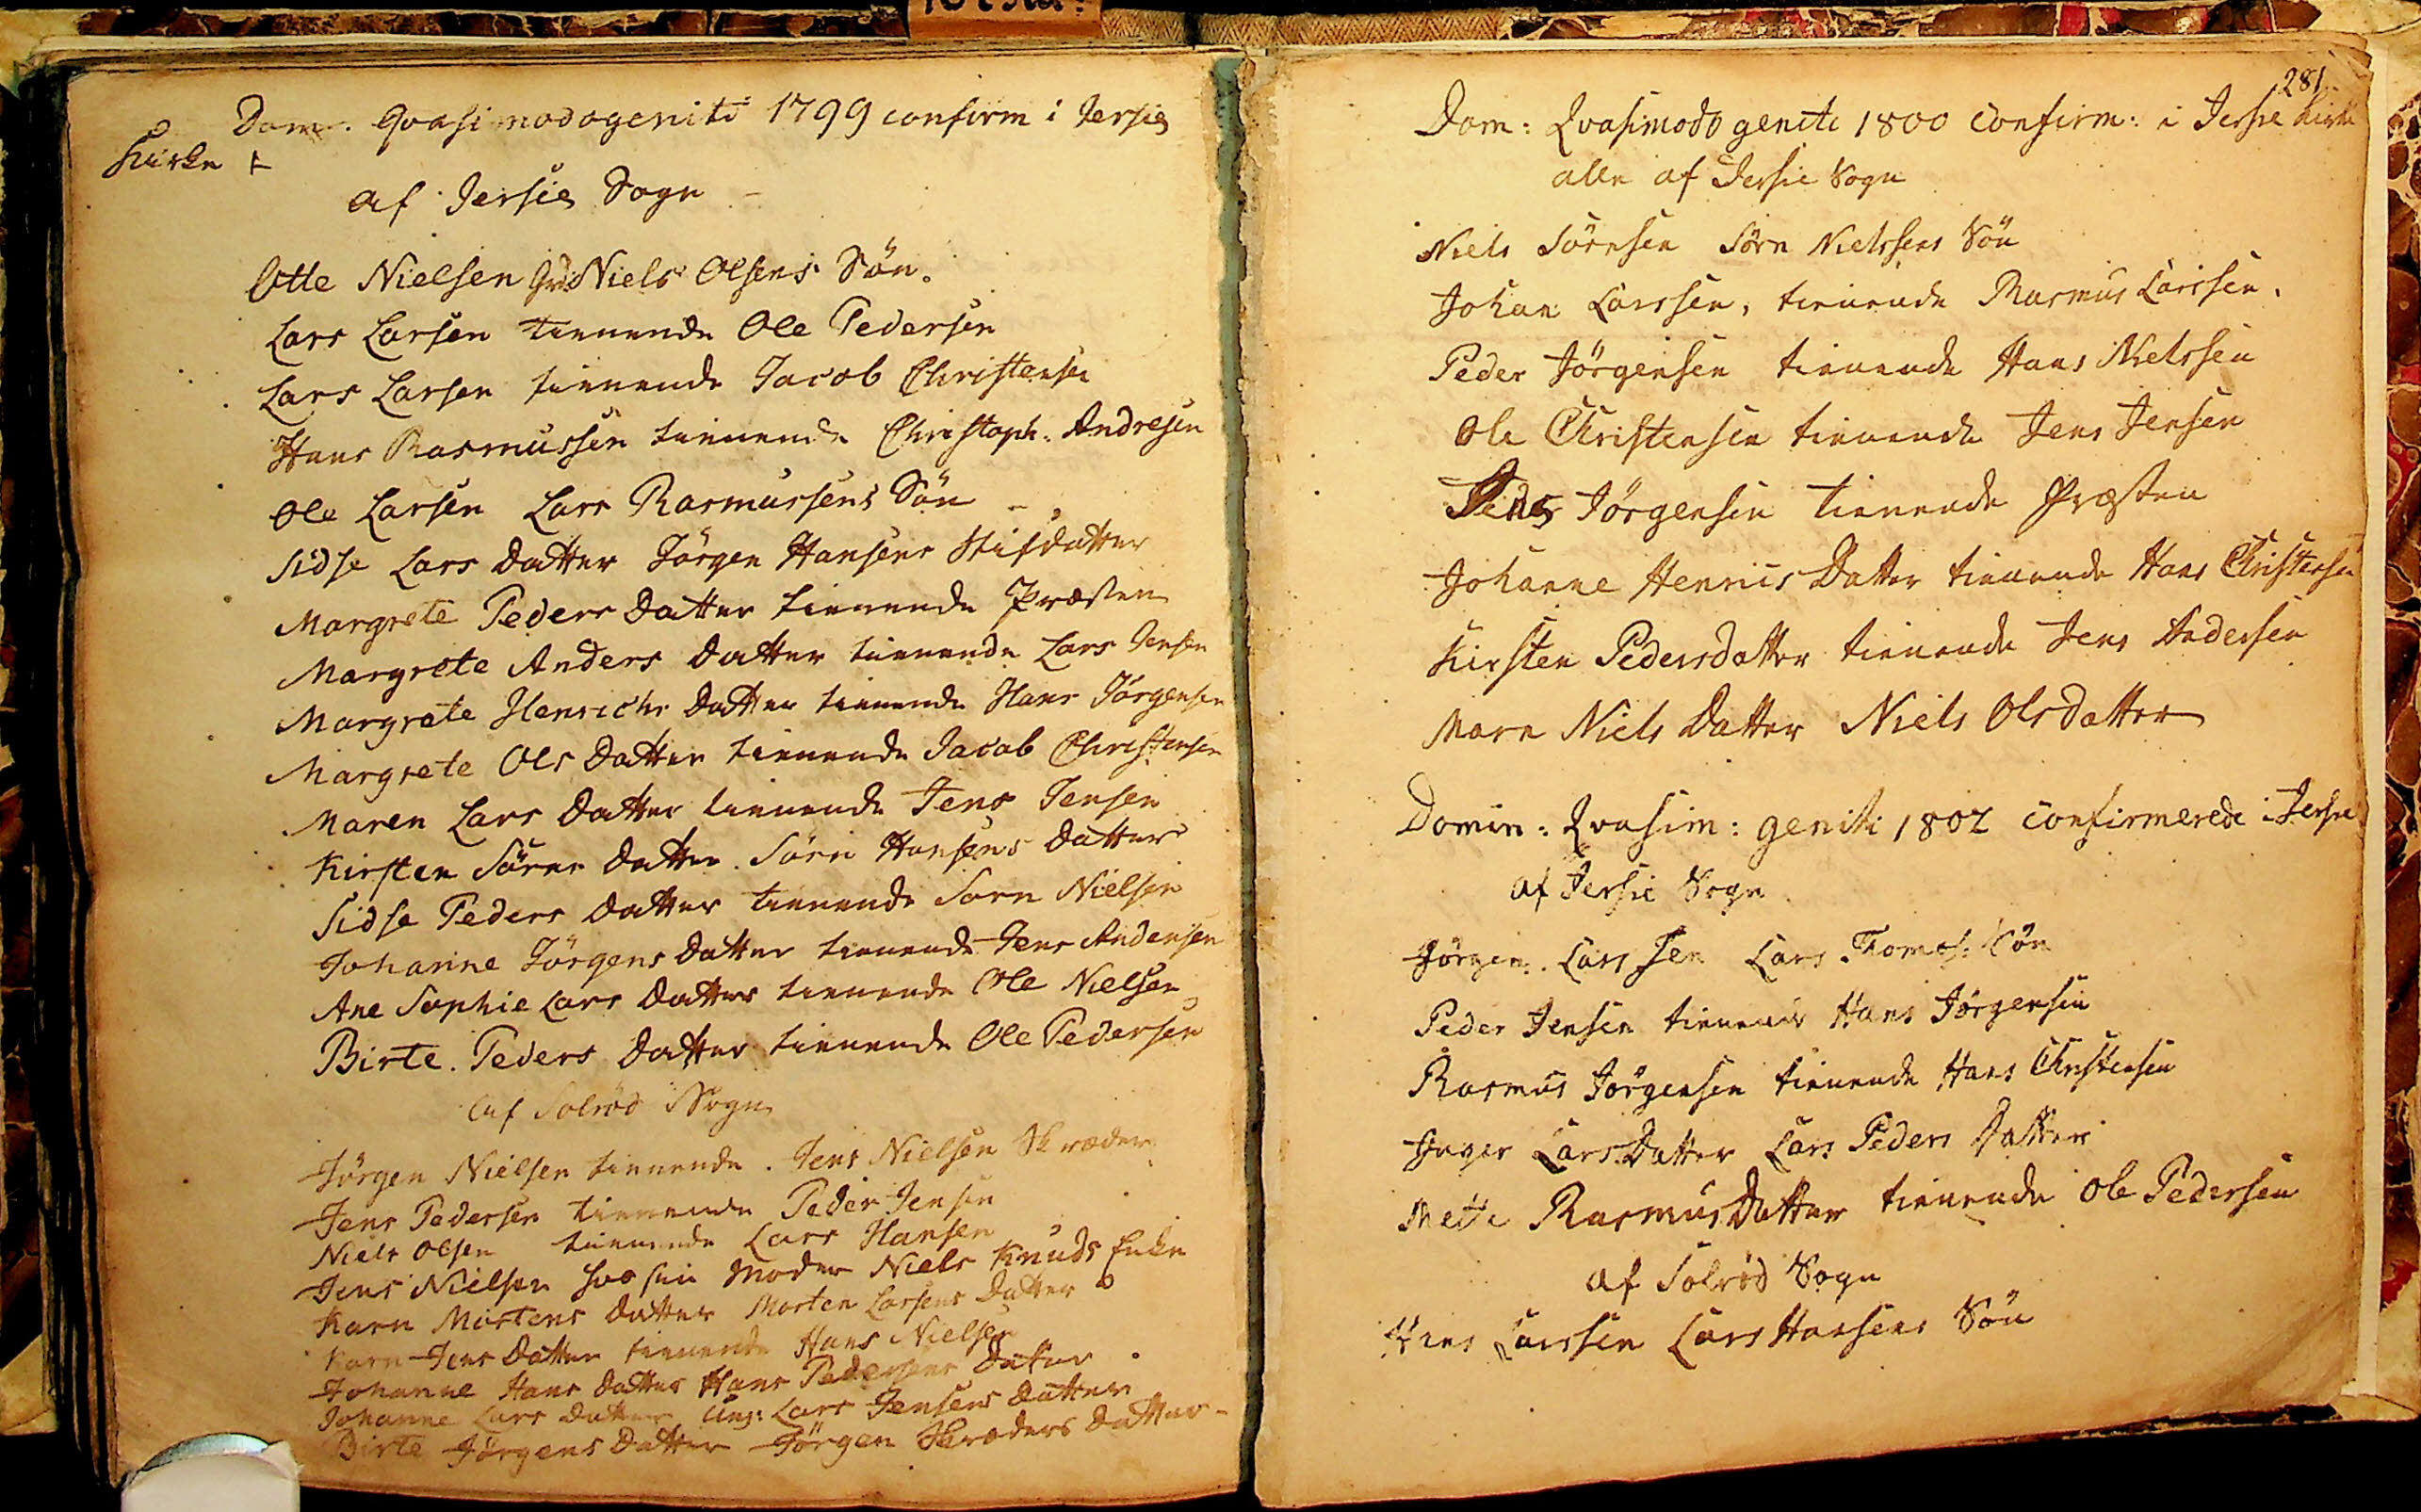Dom: Qvasimodogeniti 1799 confirm i Jersie
Kirke
Af Jersie Sogn
Otte Nielsen Gmd: Niels Olsens Søn.
Lars Larsen tienende Ole Pedersen
Lars Larsen tienende Jacob Christensen
Hans Rasmussen tienende Christoph: Andresen
Ole Larsen Lars Rasmussens Søn
Sidse Lars Datter. Jørgen Hansens Stifdatter
Margrete Peders Datter tienende Præsten
Margrete Anders Datter tienende Lars Jensen
Margrete Henrichs Datter tienende Hans Jørgensen
Margrete Oles Datter tienende Jacob Christensen
Maren Lars Datter tienende Jens Jensen
Kirsten Sørens Datter. Sørn Hansens Datter
Sidse Peders Datter tienende Sørn Nielsen
Johanne Jørgens Datter tienende Jens Andersen
Ane Sophie Lars Datter tienende Ole Nielsen
Birte Peders Datter tienende Ole Pedersen
Af Solrød Sogn
Jørgen Nielsen tienende Jens Nielsen Skræder
Jens Pedersen tienende Peder Jensen
Niels Olsen tienende Lars Hansen
Jens Nielsen hos sin Moder Niels Knuds Enke
Karn Mortens Datter Morten Larsens Datter
Karn Jens Datter tienende Hans Nielsen
Johanne Hans Datter Hans Pedersens Datter
Johanne Lars Datter Ung: Lars Jensens Datter
Birte Jørgens Datter Jørgen Skræders Datter.
281.
Dom: Qvasimodo geniti 1800 Confirm: i Jersie Kirke
alle af Jersie Sogn
Niels Sørnsen Sørn Nielssens Søn
Johan Larssen, tienende Rasmus Larssen
Peder Jørgensen tienende Hans Nielssen
Ole Christensen tienende Jens Jensen
Jens Jørgensen tienende Præsten
Johanne Henrics Datter tienende Hans Christensen
Kirsten Pedersdatter tienende Jens Andersen
Marn Niels Datter Niels Ols datter
Domin: Qvasim: Geniti 1802 Confirmerede i Jersie
af Jersie Sogn
Jørgen Larssen Lars Thomæs: Søn
Peder Jensen tienende Hans Jørgensen
Rasmus Jørgensen tienende Hans Christensen
Inger Lars Datter Lars Peders Datter
Mette Rasmus Datter tienende Ole Pedersen
Af Solrød
Hans Larssen Lars Hansens Søn
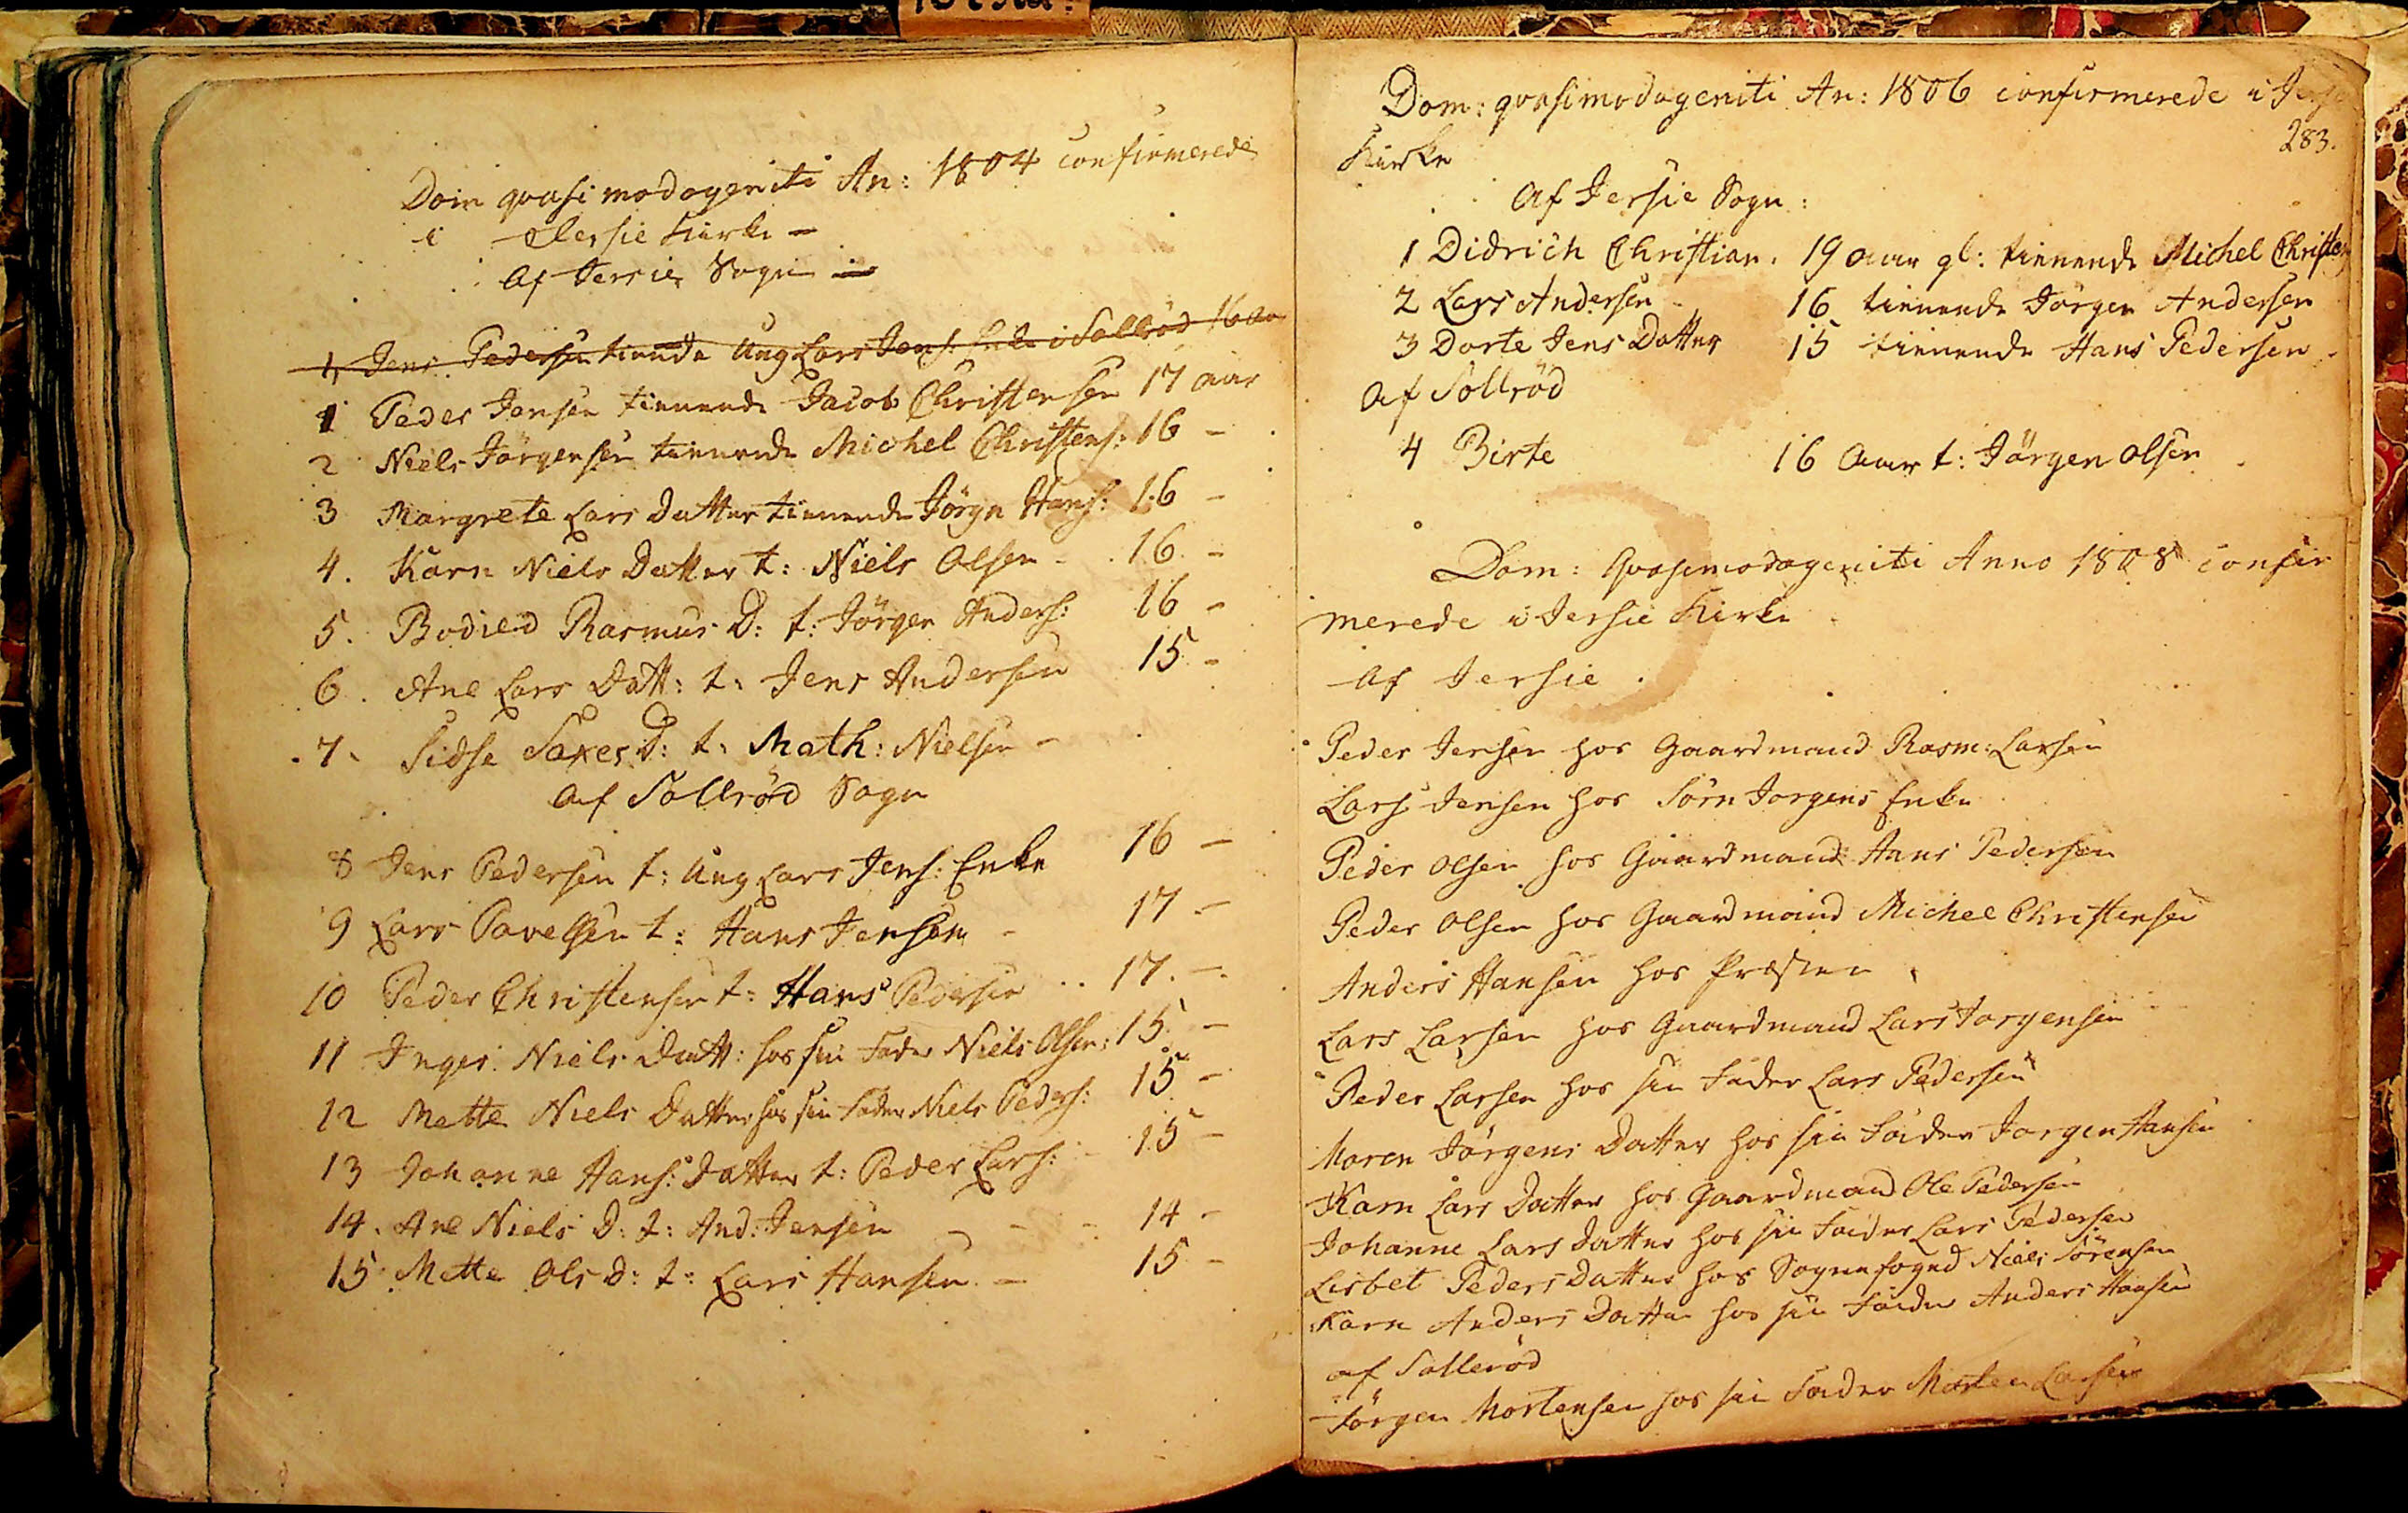Dom Qvasimodogeniti An: 1804 Confirmerede
i Jersie Kirke -
Af Jersie Sogn -
1. Jens Pedersen tiende Ung Lars Jens: Enke i Sollrød 16 Aar
1 Peder Jensen tienende Jacob Christensen 17 Aar
2 Niels Jørgensen tienende Michel Christens: 16 -
3. Margrete Lars Datter tienende Jørgn Hans: 16
4. Karn Niels Datter t: Niels Olsen 16 -
5. Bodild Rasmus D: t: Jørgen Anders: 16 -
6. Ane Lars Datt: t: Jens Andersen 15 -
7. Sidse Saxes D: t: Math: Nielsen
Af Sollrød Sogn
8 Jens Pedersen t: Ung Lars Jens: Enke 16 -
9 Lars Povelsen t: Hans Jensen 17 -
10 Peder Christensen t: Hans Pedersen 17 -
11 Inger Niels Datt: hos sin Fader Niels Olsen 15 -
12 Mette Niels Datter hos sin Fader Niels Peders: 15 -
13 Johanne Hans: Datter t: Peder Lars: 15 -
14. Ane Niels D: t: And: Jensen 14 -
15. Mette Ols D: t: Lars Hansen 15 -
283.
Dom: Qvasimodogeniti An: 1806 confirmerede i Jersie
Kirke
Af Jersie Sogn:
1 Didrich Christian: 19 Aar gl: tienende Michel Christens
2 Lars Andersen.
16 tienende Jørgen Andersen
3 Dorte Jens Datter
15 tienende Hans Pedersen
Af Sollrød
4 Birte
16 Aar t: Jørgen Olsen
Dom: Qvasimodogeniti Anno 1808 confir¬
merede i Jersie Kirke
Af Jersie
Peder Jensen hos Gaardmand Rasm: Larsen
Lars Jensen hos Sørn Jørgens Enke
Peder Olsen hos Gaardmand Hans Pedersen
Peder Olsen hos Gaardmand Michel Christensen
Anders Hansen hos Præsten
Lars Larsen hos Gaardmand Lars Jorgensen
Peder Larsen hos sin Fader Lars Pedersen
Maren Jørgens Datter hos sin Fader Jorgen Hansen
Karn Lars Datter hos Gaardmand Ole Pedersen
Johanne Lars Datter hos sin Fader Lars Pedersen
Lisbet Peders Datter hos Sognefoged Niels Sørensen
Karn Anders Datter hos sin Fader Anders Hansen
af Sollerød
Jørgen Mortensen hos sin Fader Morten Larsen